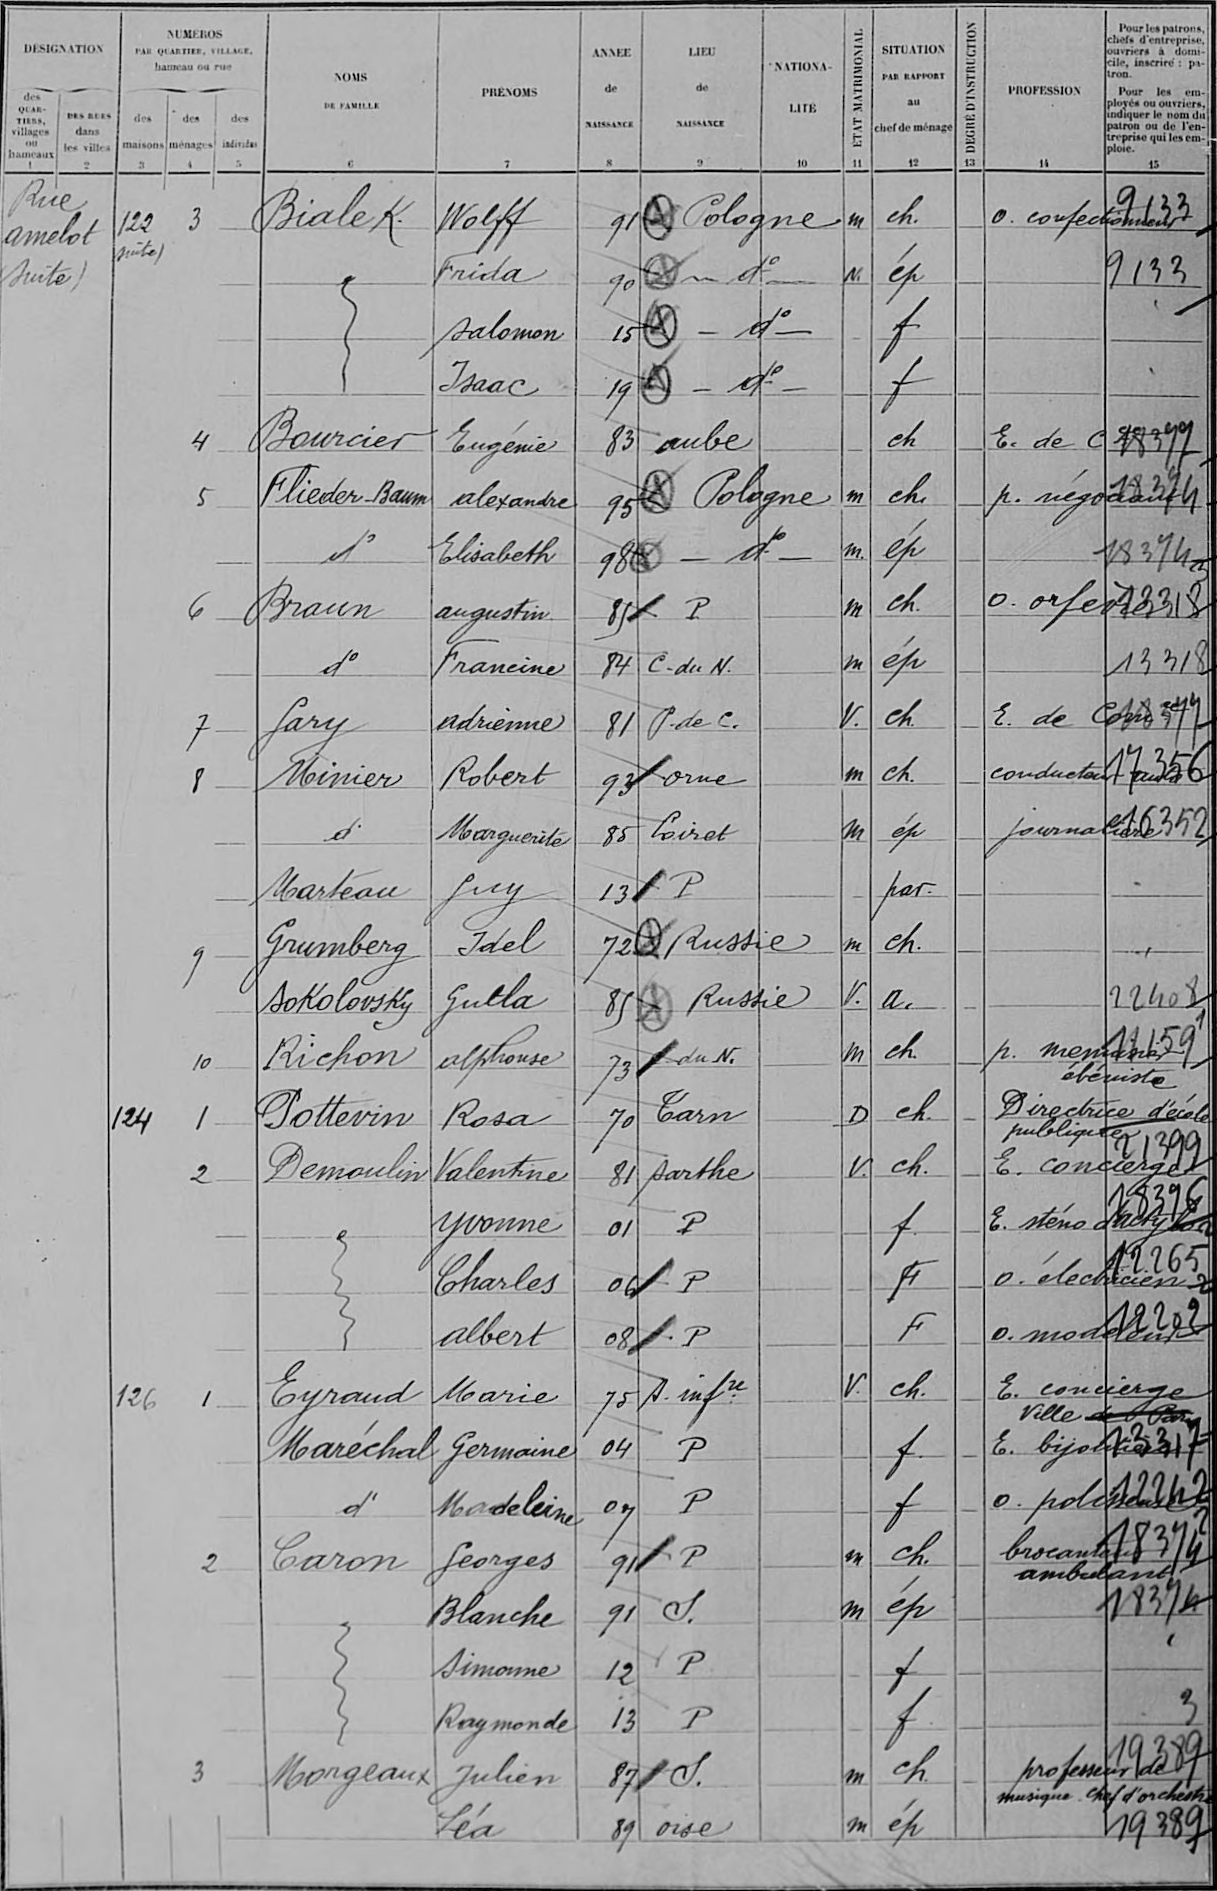Bialek/Wolff/91/Cologne/¤/m/ch /¤/O confectionneur/9133
¤/Frida/90/d°/¤/M/ép/¤/¤/9133
¤/Salomon/15/d°/¤/¤/f/¤/¤/¤
¤/Isaac/19/d°/¤/¤/f/¤/¤/¤
Bourcier/Eugénie/83/aube/¤/¤/ch/¤/E de C/18377
Flieder Baum/Alexandre/95/Pologne/¤/m/ch/¤/p négociant/18374
d°/Elisabeth/98/d°/¤/m/ép/¤/¤/183743
Braun/Augustin/85/P/¤/m/ch /¤/o orfèvre/13318
d°/Francine/84/C du N /¤/m/ép/¤/¤/13318
Jary/Adrienne/81/P de C /¤/V /ch/¤/E de Comce/18377
Moinier/Robert/93/Orne/¤/m/ch /¤/conducteur auto/17356
d°/Marguerite/85/Loiret/¤/m/ép/¤/journalière/16352
Marteau/Lucy/13/P/¤/¤/par /¤/¤/¤
Grumberg/Idel/72/Russie/¤/m/ch /¤/¤/¤
Sokolovsky/Gutla/85/Russie/¤/V /a /¤/¤/22408
Richon/alphonse/73/C du N /¤/m/ch/¤/p menuisier! ebeniste/11159
Pottevin/Rosa/70/Tarn/¤/D/ch /¤/directrice d'école! publique/!21399
Demoulin/Valentine/81/Sarthe/¤/V /ch /¤/E concierge/!18396
¤/Yvonne/01/P/¤/¤/f /¤/E sténo dactylo/?18396
¤/Charles/06/P/¤/¤/F/¤/o électricien/?12263
¤/albert/08/P/¤/¤/F/¤/o modeleur/?12202
Eyraud/Marie/75/S infre/¤/V /ch /¤/E concierge! ville de Paris/¤
Maréchal/Germaine/04/P/¤/¤/f /¤/E bijoutière/12317
d°/Madeleine/07/P/¤/¤/f/¤/o polisseur/12242
Caron/Georges/91/P/¤/m/ch /¤/brocanteur! ambulant/18374
¤/Blanche/91/S /¤/m/ép/¤/¤/18374
¤/Simonne/12/P/¤/¤/f/¤/¤/¤
¤/Raymonde/13/P/¤/¤/f/¤/¤/3
Morgeaux/Julien/87/S /¤/m/ch/¤/professeur de! musique chef d'orchestre/19389
¤/Léa/89/oise/¤/m/ép/¤/¤/19389
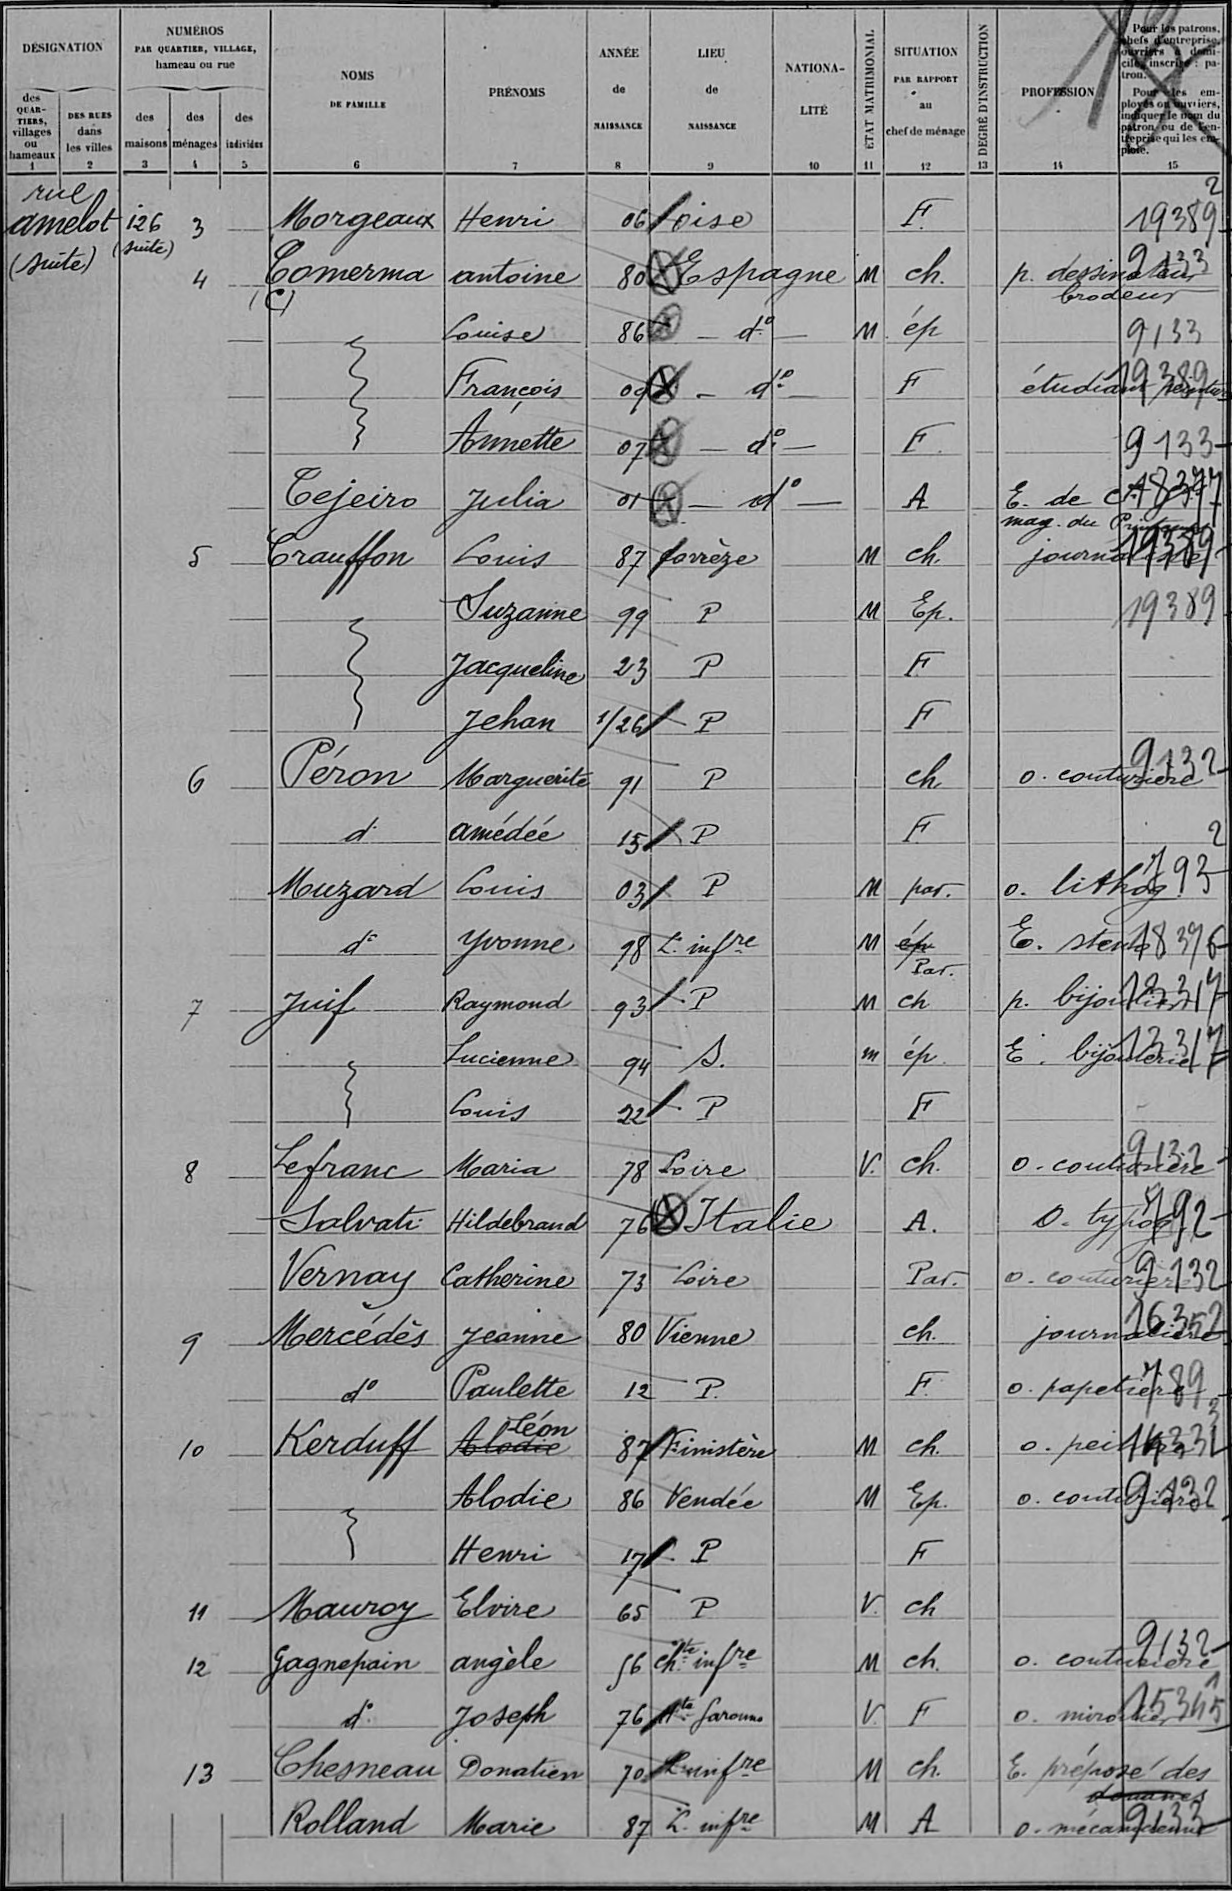Morgeaux/Henri/06/Oise/¤/¤/F /¤/¤/19389
Comerma/antoine/80/Espagne/¤/M/ch /¤/p dessinateur!brodeur/9133
¤/Louise/86/d°/¤/M/ép/¤/¤/9133
¤/François/09/d°/¤/¤/F/¤/étudiant peinture/?19389
¤/Annette/07/d°/¤/¤/F/¤/¤/9133
Cejeiro/Julia/01/d°/¤/¤/A/¤/E de C mag du Printemps/?18377
Crauffon/Louis/87/Corrèze/¤/M/ch /¤/journaliste/19389
¤/Suzanne/99/P/¤/M/Ep /¤/¤/19389
¤/Jacqueline/23/P/¤/¤/F/¤/¤/¤
¤/Jehan/126/P/¤/¤/F/¤/¤/¤
Péron/Marguerite/91/P/¤/¤/ch/¤/o couturiere/?9132
d/amédée/15/P/¤/¤/F/¤/¤/2
Mouzard/Louis/03/P/¤/M/par /¤/o lithog/?793
d°/Yvonne/98/L Infre/¤/M/ép Par /¤/E sténo/18376
Juif/Raymond/93/P/¤/M/ch/¤/p bijouterie/?13317
¤/Lucienne/94/S /¤/m/ép/¤/E bijouterie/?13317
¤/Louis/22/P/¤/¤/F/¤/¤/¤
Lefranc/Maria/78/Loire/¤/V /ch /¤/o couturière/?9132
Salvati/Hildebrand/76/Italie/¤/¤/A /¤/O typog/792
Vernay/Catherine/73/Loire/¤/¤/Par /¤/O couturière/?9132
Mercédès/Jeanine/80/Vienne/¤/¤/ch /¤/journalière/16352
d°/Paulette/12/P /¤/¤/F /¤/o papetier/789
Kerduff/Alodie Léon/87/Finistère/¤/M/ch /¤/o peintre/?14331
¤/Alodie/86/Vendée/¤/M/Ep /¤/o couturière/?9132
¤/Henri/17/P/¤/¤/F/¤/¤/¤
Mauroy/Elvire/65/P/¤/V /ch/¤/¤/¤
gagnepain/Angèle/56/chte infre/¤/M/ch/¤/o couturier/?9132
d°/Joseph/76/Hte Garonne/¤/V/F/¤/o miroitier/15345 1
Chesneau/Donatien/70/L Infre/¤/M/ch /¤/E preposé des !douanes/¤
Rolland/Marie/87/L Infre/¤/M/A/¤/o mécanicienne/?9133